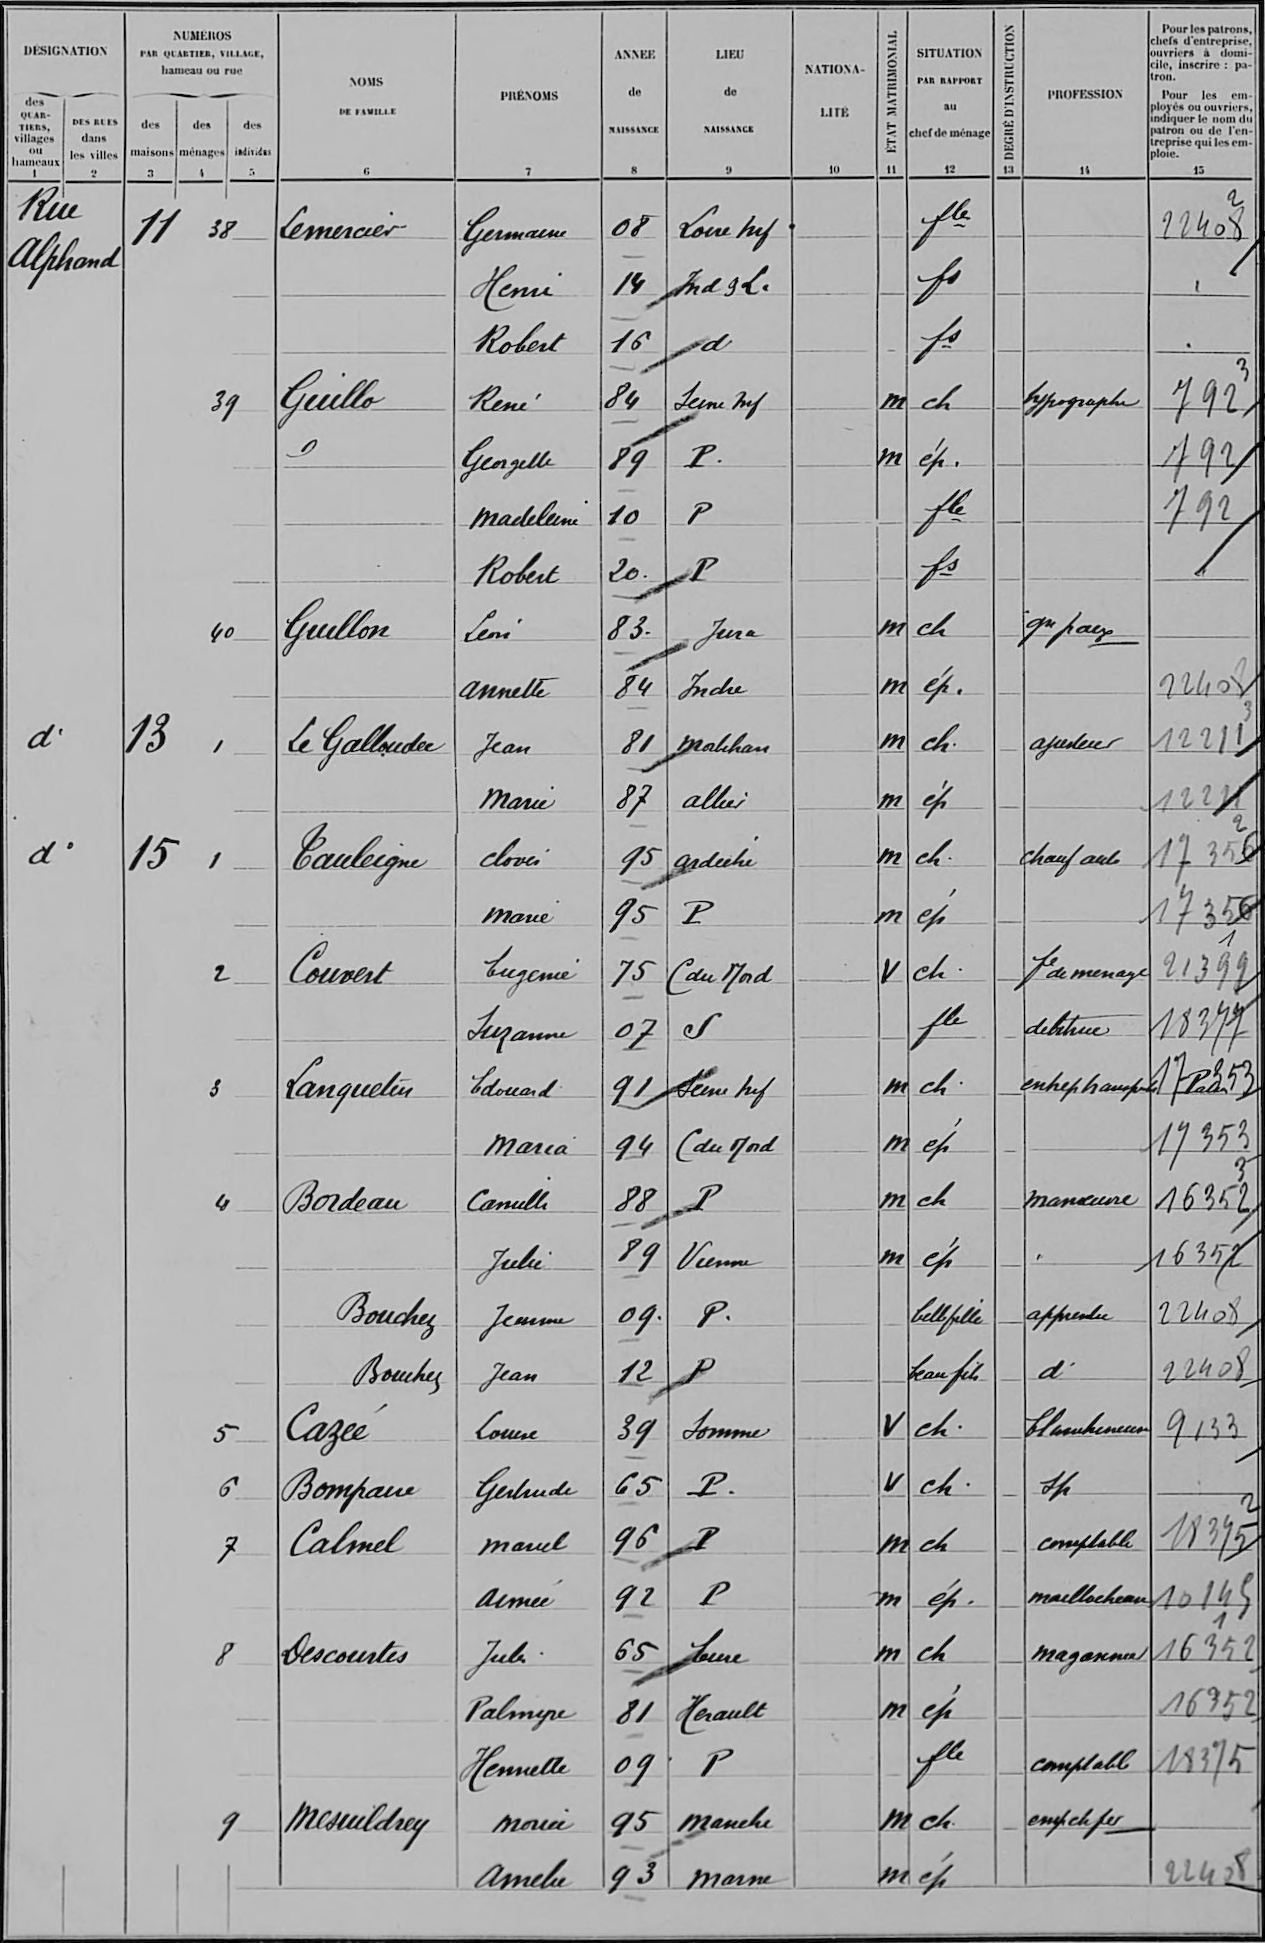Lemercier/Germaine/08/Loire Inf/¤/¤/fle/¤/¤/22408
¤/Henri/14/Ind L /¤/¤/fs/¤/¤/¤
¤/Robert/16/d/¤/¤/fs/¤/¤/¤
Guillo/René/84/Seine Inf/¤/m/ch/¤/typographe/792
¤/Georgette/89/P /¤/m/ép /¤/¤/792
¤/Madeleine/10/P/¤/¤/fle/¤/¤/792
¤/Robert/20 /P/¤/¤/fs/¤/¤/¤
Guillon/Leon/83/Jura/¤/m/ch/¤/gn paix/¤
¤/Annette/84/Indre/¤/m/ép /¤/¤/22403
Le Galloudec/Jean/81/Morbihan/¤/m/ch /¤/ajusteur/12281
¤/Marie/87/Allier/¤/m/ép/¤/¤/12211
Cauleigne/Clovis/95/Ardeche/¤/m/ch /¤/chauf auto/17356
¤/Marie/95/P/¤/m/ép/¤/¤/17356
Couvert/Eugenie/75/C du Nord/¤/V/ch /¤/f de menage/21399
¤/Suzanne/07/S/¤/¤/fle/¤/debitrice/18377
Lanquelin/Edouard/91/Seine Inf/¤/m/ch/¤/entrep transports/Paris?17353
¤/Maria/94/C du Nord/¤/m/ep/¤/¤/173533
Bordeau/Camille/88/P/¤/m/ch/¤/manoeuvre/16352
¤/Julie/89/Vienne/¤/m/ép/¤/¤/16352
Bouchez/Jeanne/09 /P /¤/¤/bell fille/¤/apprentie/22408
Bouchez/Jean/12/P/¤/¤/beau fils/¤/d°/22408
Cazée/Louise/39/Somme/¤/V/ch /¤/Blanchisseuse/9133
Bompaire/Gertrude/65/P /¤/V/ch /¤/spr/2
Calmel/Mariel/96/P/¤/m/ch/¤/comptable/18375
¤/Aimée/92/P/¤/m/ép /¤/maillocheuse/10145
Descourtes/Jules/65/Eure/¤/m/ch/¤/magasinier/16352
¤/Palmyre/81/Herault/¤/m/ép/¤/¤/16352
¤/Henriette/09/P/¤/¤/fle/¤/comptable/18375
Mesuildrey/Morice/95/Manche/¤/m/ch/¤/emp ch fer/¤
¤/Amelie/93/Marne/¤/m/ép/¤/¤/22428
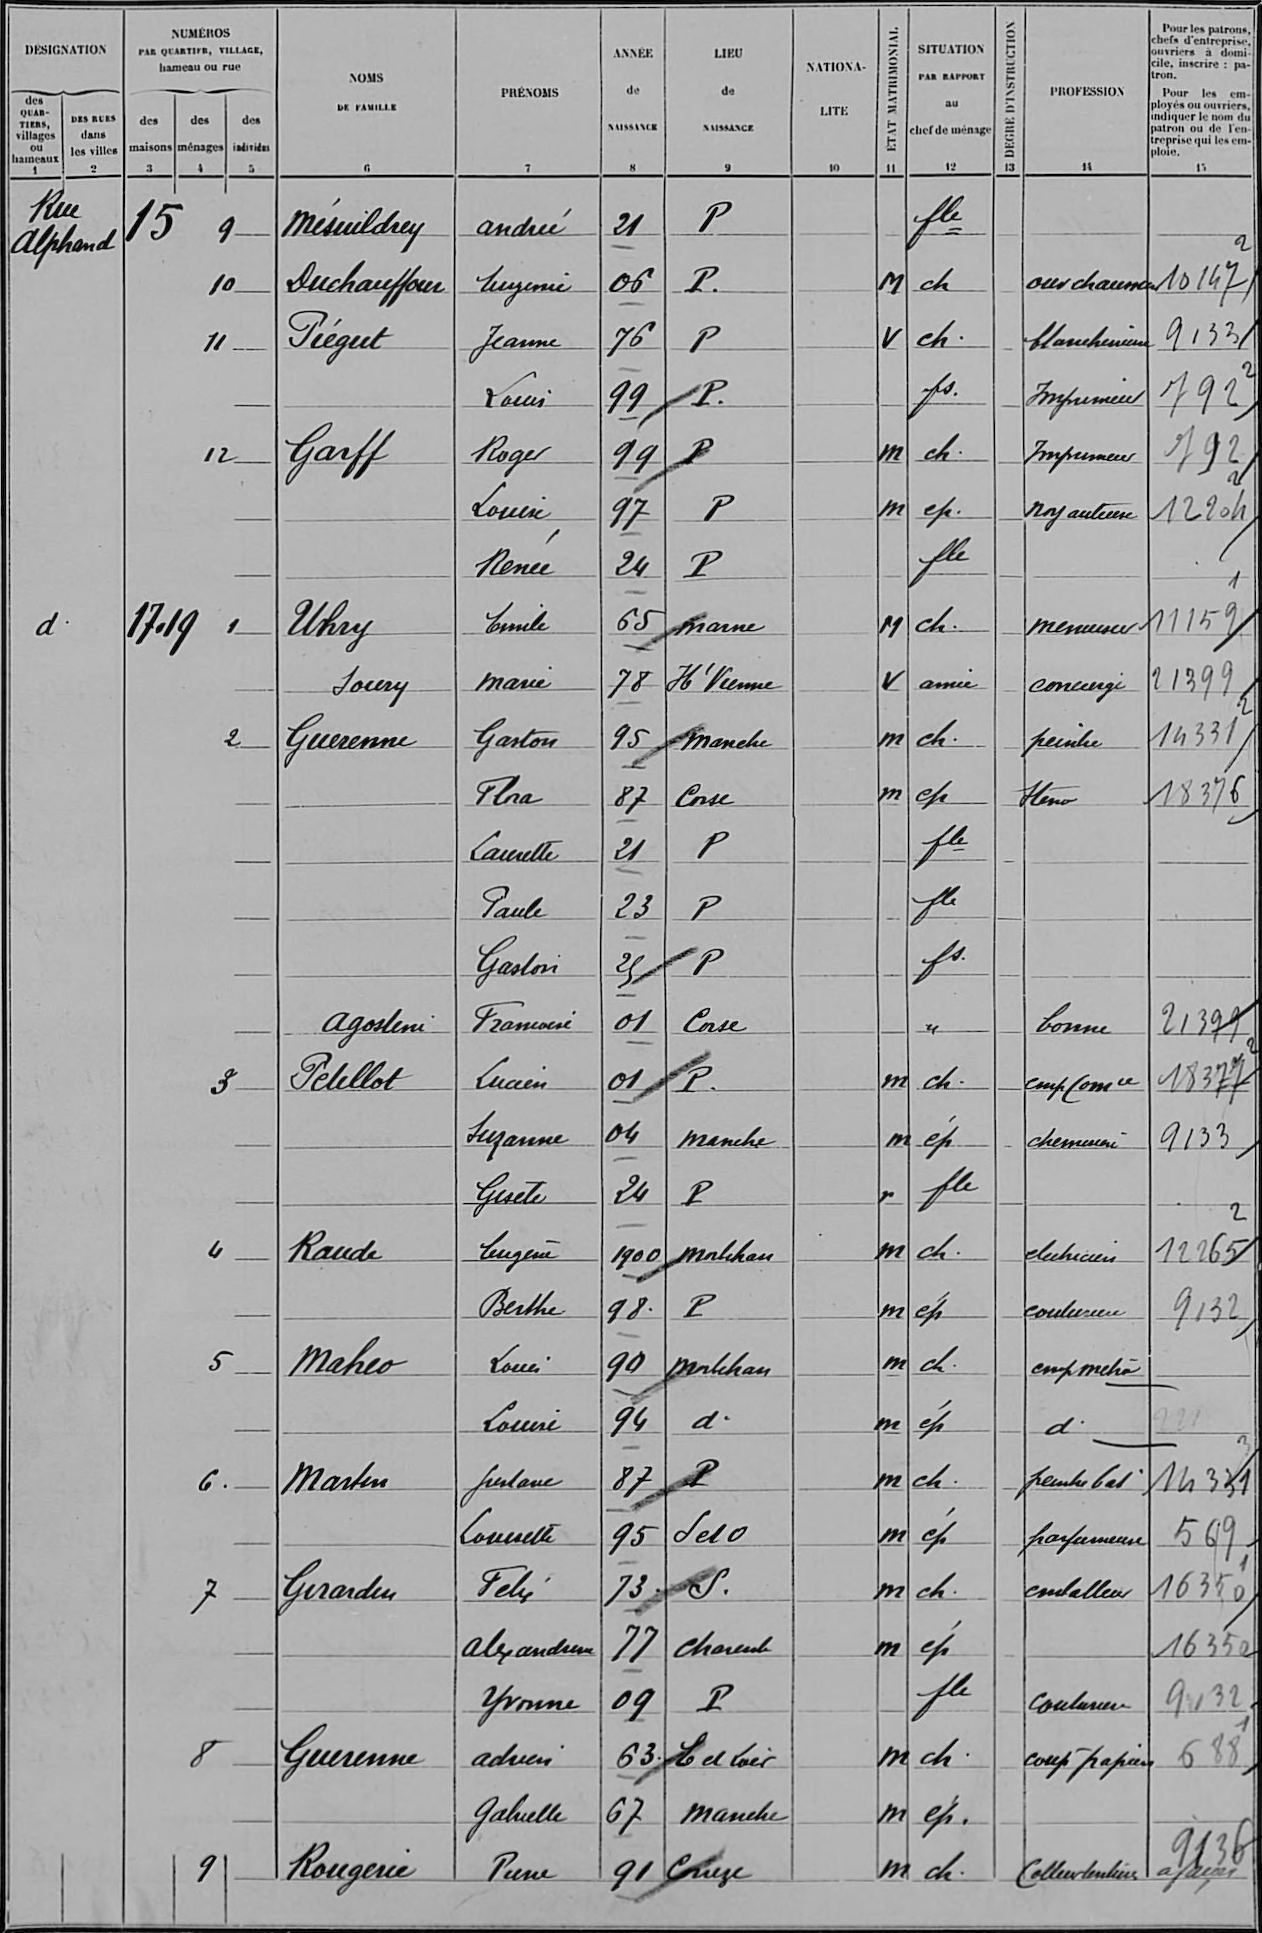Mésuildrey/Andrée/21/P/¤/¤/fle/¤/¤/¤
Duchauffour/Eugénie/06/P /¤/M/ch/¤/ouv chausseur/10147
Piégut/Jeanne/76/P/¤/V/ch /¤/blanchisserie/9133
¤/Louis/99/P /¤/¤/fs/¤/Imprimeur/7922
Garf/Roger/99/P/¤/m/ch /¤/Imprimeur/7922
¤/Louise/97/P/¤/m/ep /¤/Noyauteuse/12204
¤/Renée/24/P/¤/¤/flle/¤/¤/¤
Uhry/Emile/65/Marne/¤/M/ch /¤/menuisier/11159
Soury/Marie/78/Ht Vienne/¤/V/amie/¤/concierge/21399
Guerenne/Gaston/95/Manche/¤/m/ch /¤/peintre/14331
¤/Flora/87/Corse/¤/m/ep/¤/Steno/18376
¤/Laurette/21/P/¤/¤/fle/¤/¤/¤
¤/Paule/23/P/¤/¤/fle/¤/¤/¤
¤/Gaston/25/P/¤/¤/fs/¤/¤/¤
Agostini/Francoise/01/Corse/¤/¤/¤/¤/bonne/21399
Petillot/Lucien/01/P /¤/m/ch /¤/emp Comce/18377
¤/Suzanne/04/Manche/¤/m/ép/¤/chemisiere/9133
¤/Gisèle/24/P/¤/v/fle/¤/¤/¤
Raude/Eugène/1900/Morbihan/¤/m/ch /¤/electricien/12265
¤/Berthe/98/P/¤/m/ép/¤/couturiere/9132
Maheo/Louis/90/Morbihan/¤/m/ch /¤/emp métro/¤
¤/Louise/94/d°/¤/m/ép/¤/d°/¤
Martin/Gustave/87/P/¤/m/ch /¤/peintre bat/14331
¤/Louisette/95/S et O/¤/m/ép/¤/parfumeuse/569
Girardin/Felix/73/S /¤/m/ch /¤/emballeur/16350
¤/Alexandrine/77/Charente/¤/m/ép/¤/¤/16352
¤/Yvonne/09/P/¤/¤/fle/¤/couturier/9132
Guerenne/Adrien/63/E et Loir/¤/m/ch/¤/coup papiers/688
¤/Gabrielle/67/Manche/¤/m ép /¤/¤/¤/¤
Rougerie/Pierre/91/Corrèze/¤/m/ch /¤/Colleurdentieur/à façon?9136